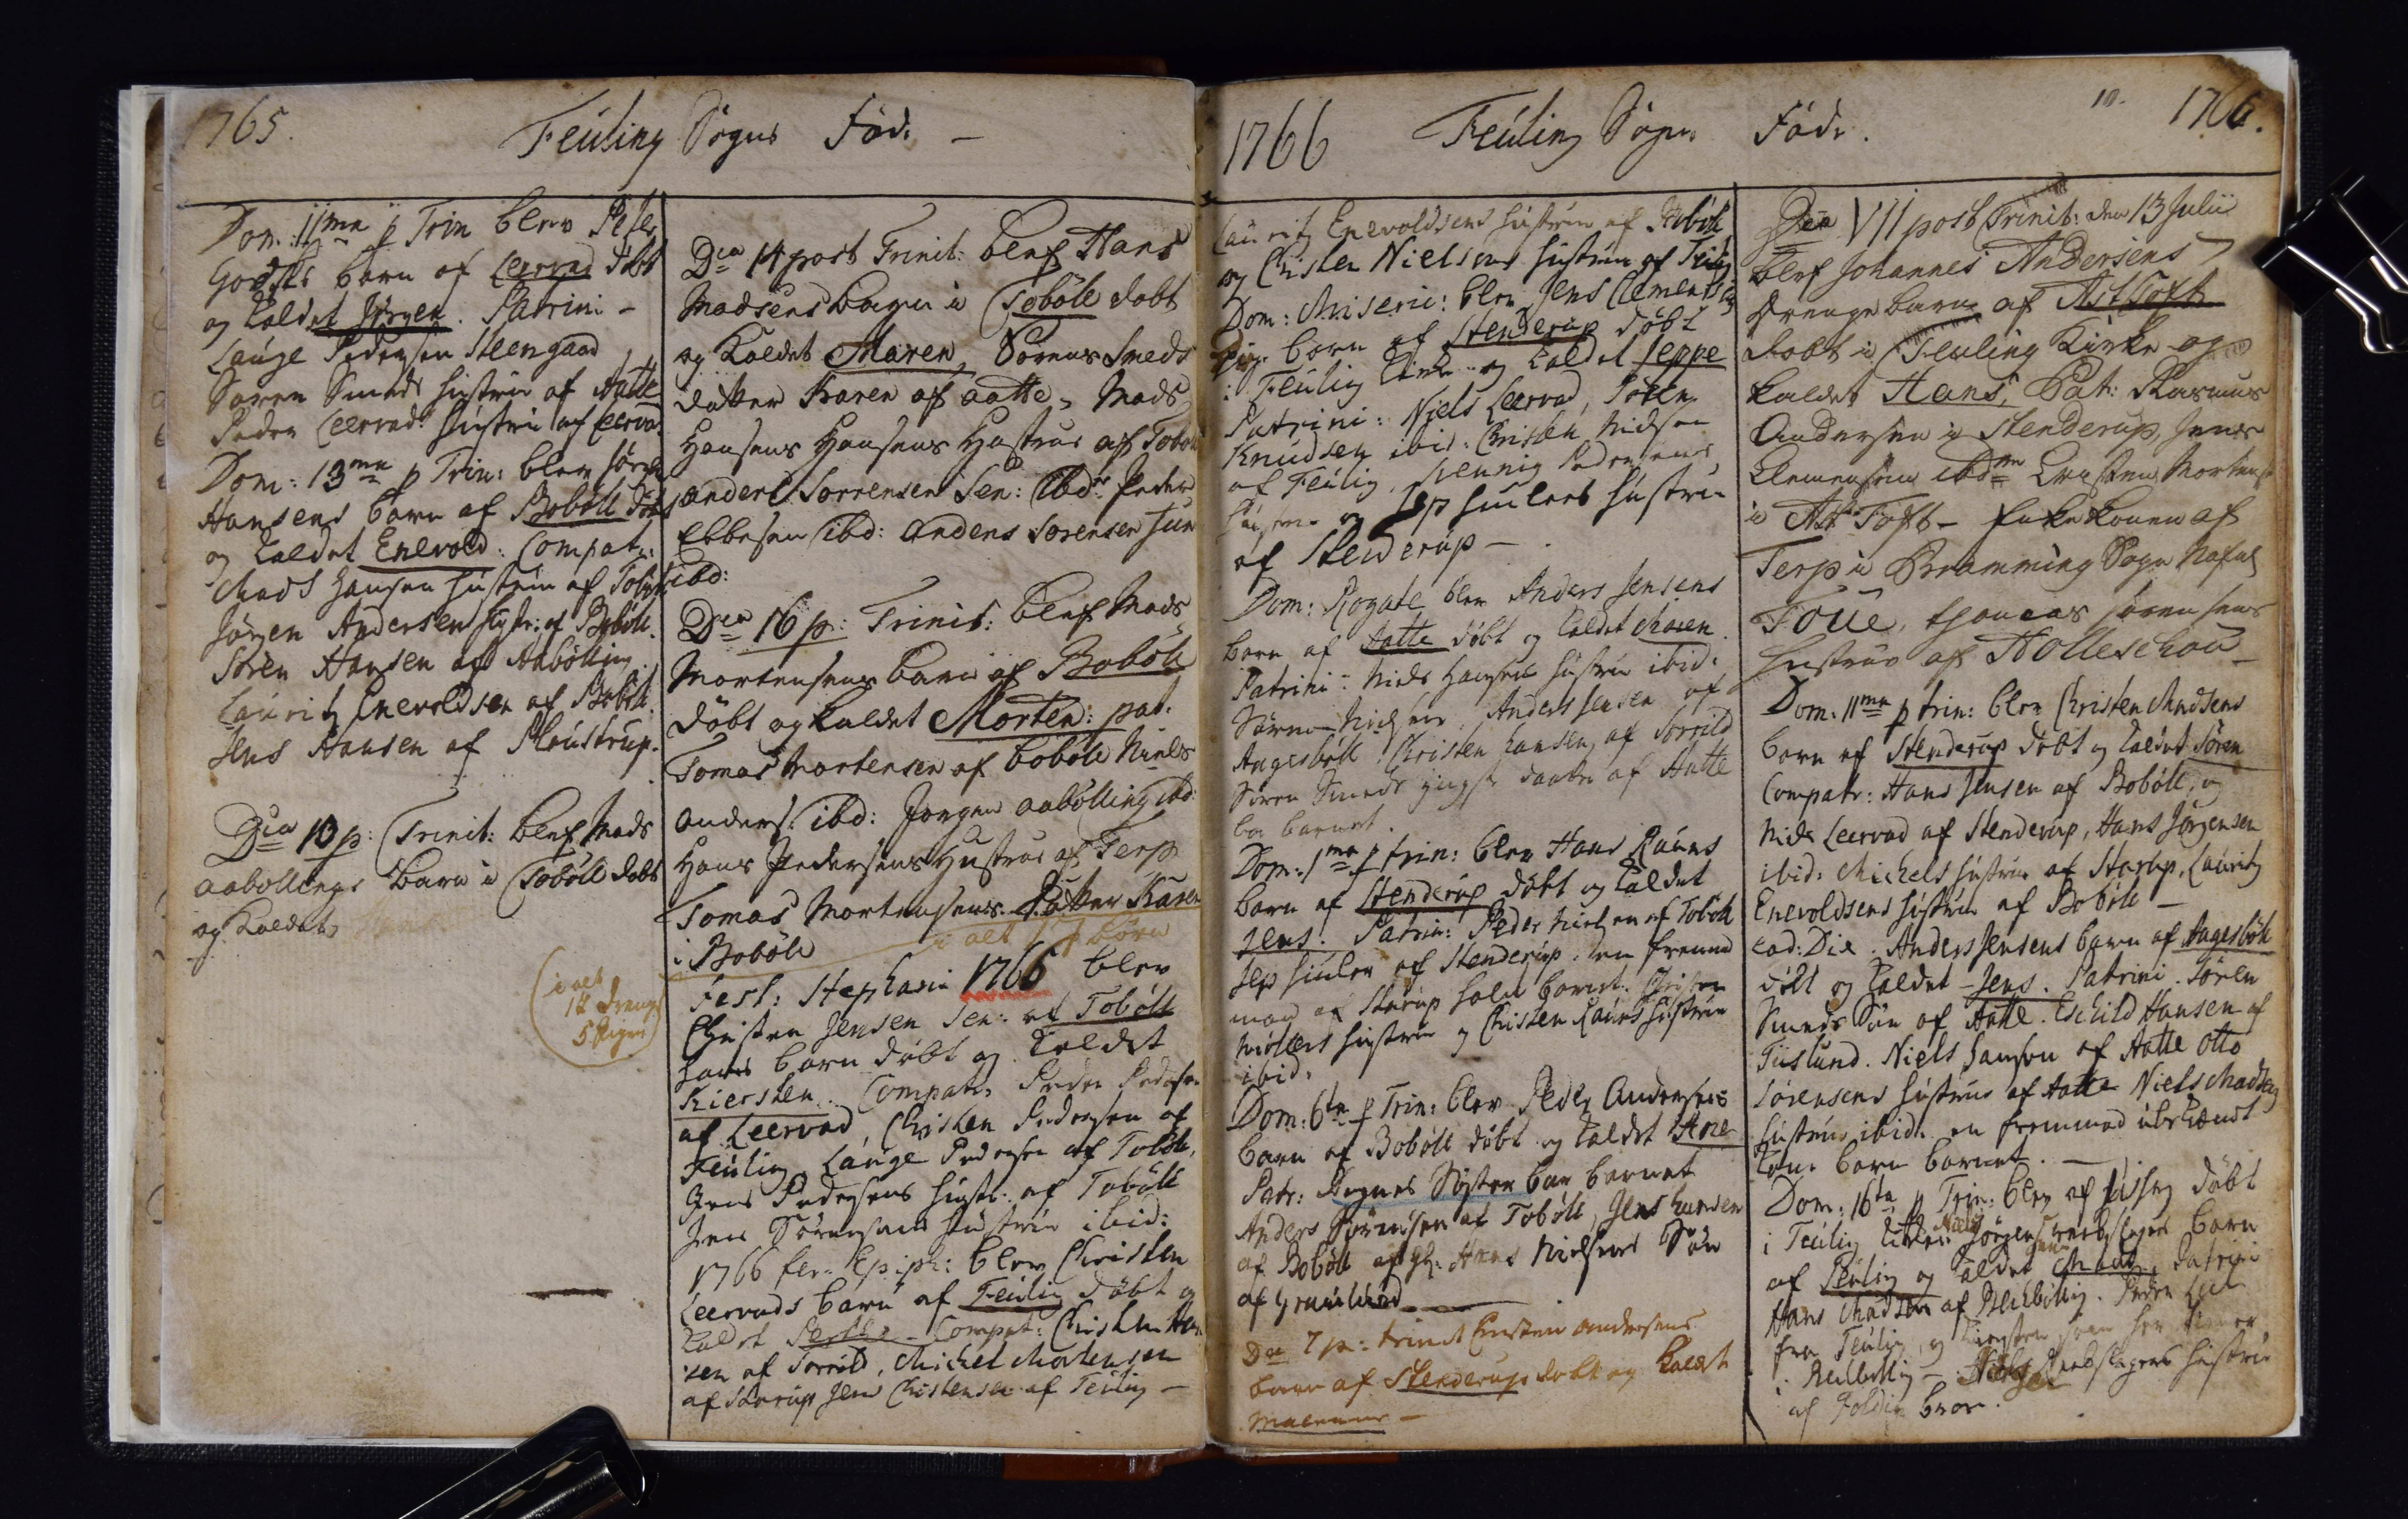1765.
Feuling Sogns Fød:
Dom: 11ma p Trin blev Peter
Godsks Barn af Leervad døbt
og kaldet Jørgen. Patrini -
Lauge Pedersen Steengaard
Søren Smeds hustru af Aatte
Peder Leervads hustru af Leervad
Dom: 13ma p Trin: blev Jørgen
Hansens barn af Bobøll døbt
og kaldet Enevold: Compat:
Mads hansen hustrue af Tobøl
Jørgen Andersens hustr: af Bobøll.
Søren Hansen af Aabølling
Lauritz Enevoldsen af Bobøl
Jens Hansen af Ploustrup.
Dca 10 p: Trinit: blef Mads
Aabøllings barn i Tobøll døbt
og kaldet
i alt
17 Drenge
5 Piger
Dca 14 post Trinit: blef Hans
Madsens barn i Tobøll dobt
og kaldet Maren, Sørens Smeds
datter Karen af Aatte, Mads
Hansens Hansens Hustrue af Toboll
Anders Sørrensen Sen: ibd## Peder
Ebbesøn ibd: Anders Sørensen Jun
ibd:
Dca 16 p: Trinit: blef Mads
Mortensens barn af Bobøll
døbt og kaldet Morten: pat.
Tomas Mortensen af bobøll Niels
Anders: ibd: Jørgen Aabølling ibid:
Hans Pedersens Hustrue af Terp
Tomas Mortensens Datter Karen
i Bobøl.
i alt 17 Børn
Fest: Stephani 1766 blev
Christen Jensen Sen: af Tobøll
hans barn døbt og kaldet
Kiersten. Compatr, Peder Pedersen
af Leervad, Christen Pedersen af
Feuling, Lauge Pedersen af Tobøll,
Jens Pedersens Hustr: af Tobøll
Jens Sørrensens Hustrue ibid:
1766 Fer: Epiph: blev Christen
Leervads barn af Feuling døbt og
kaldet ##rth## Compat: Christen Han¬
sen af Sorrild, Michel Mortensen
af Storup Jens Christensen af Feuling -
1766
Feuling Sogns Føde
Lauritz Enevoldsens hustrue af Bobøll
og Christen Nielsens Hustrue af Feuling
Dom: Miseric: blev Jens Clemensens
Pige Barn af Stenderup døbt
i Feuling Kirke og kaldet Jeppe
Patrini: Niels Leervad, Søren
Knudsen ibid. Christen Nielsen
af Feuling, Hennig Pedersens
hustru og Jep hiulers hustru
af Stenderup -
Dom: Rogate blev Anders Jensens
Barn af Aatte døbt og kaldet Maren
Patrini: Niels Hansens hustru ibid:
Søren Nielsen Anders Jensen af
Aagesbøll Christen Hansen af Sorrild
Søren Smeds Hustr daatter af Aatte
bar barnet.
Dom: 1ma p Trin: blev Hans Rauns
Barn af Stenderup døbt og kaldet
Jens. Patrin: Peder Nielsen af Tobøll
Jep hiulers af Stenderup. en fremmed
mand af Starup holdt barnet. Christen
Nielsens hustrue og Christen Rauns hustrue
ibid:
Dom. 6ta p Trin: blev Peder Andersens
Barn af Bobøll døbt og kaldet Ane
Patr: Degnes Søster bar barnet
Anders Sørensen af Tobøll, Jens Larsen
af Bobøll af gl: Hans Nielsøns Søn
af Graulund.
Dom: 7 p: Trinit Christen Andersens
barn af Stenderup døbt og kaldet
Maleene
10.
1766.
Dca VII post Trinit: d 13 Julii
blef Johannes Andersens
Drengebarn af Astoft
døbt i Feuling Kirke og
kaldet Hans Pat: Rasmus
Anderssen i Stenderup, Jens
Clemensen ibdm Kristen Mortensen
i Astoft. Enke Konen af
Terp i Bramming Sogn Nafnl
Toue, Thomas Sørensens
Hustrue af Holleschou
Dom: 11ma p Trin: blev Christen Madsens
Barn af Stenderup døbt og kaldet Søren
Compatr: Hans Jensen af Bobøll, og
Niels Leerved af Stenderup, Hans Jørgensen
ibid: Michels Hustrue af Starup, Lauritz
Enevoldsens Hustrue af Bobøll -
Eod: Die: Anders Jensens barn af Aagesbøll
døbt og kaldet - Jens. Patrini. Sørn¬
Smeds Søn af Aatte Eschild Hansen af
Tiislund. Niels Hansen af Aatte Otto
Sørensens hustrue af Aatte Niels Madsens
Hustrue ibid. en fremmed ubekiændt
kone barn barnet.
Dom: 16ta p Trin: blev af ## døbt
Niels
i Feuling Kirke Jørgen reebslagers barn
Jens
af Feuling og kaldet Mads Patrini
Hans Madsen af Bechbølling. Peder L##
fra Feuling, og Kiersten ## her ##
i Bechbølling - Jørgen Reebslagers hutrue
af Folding broe
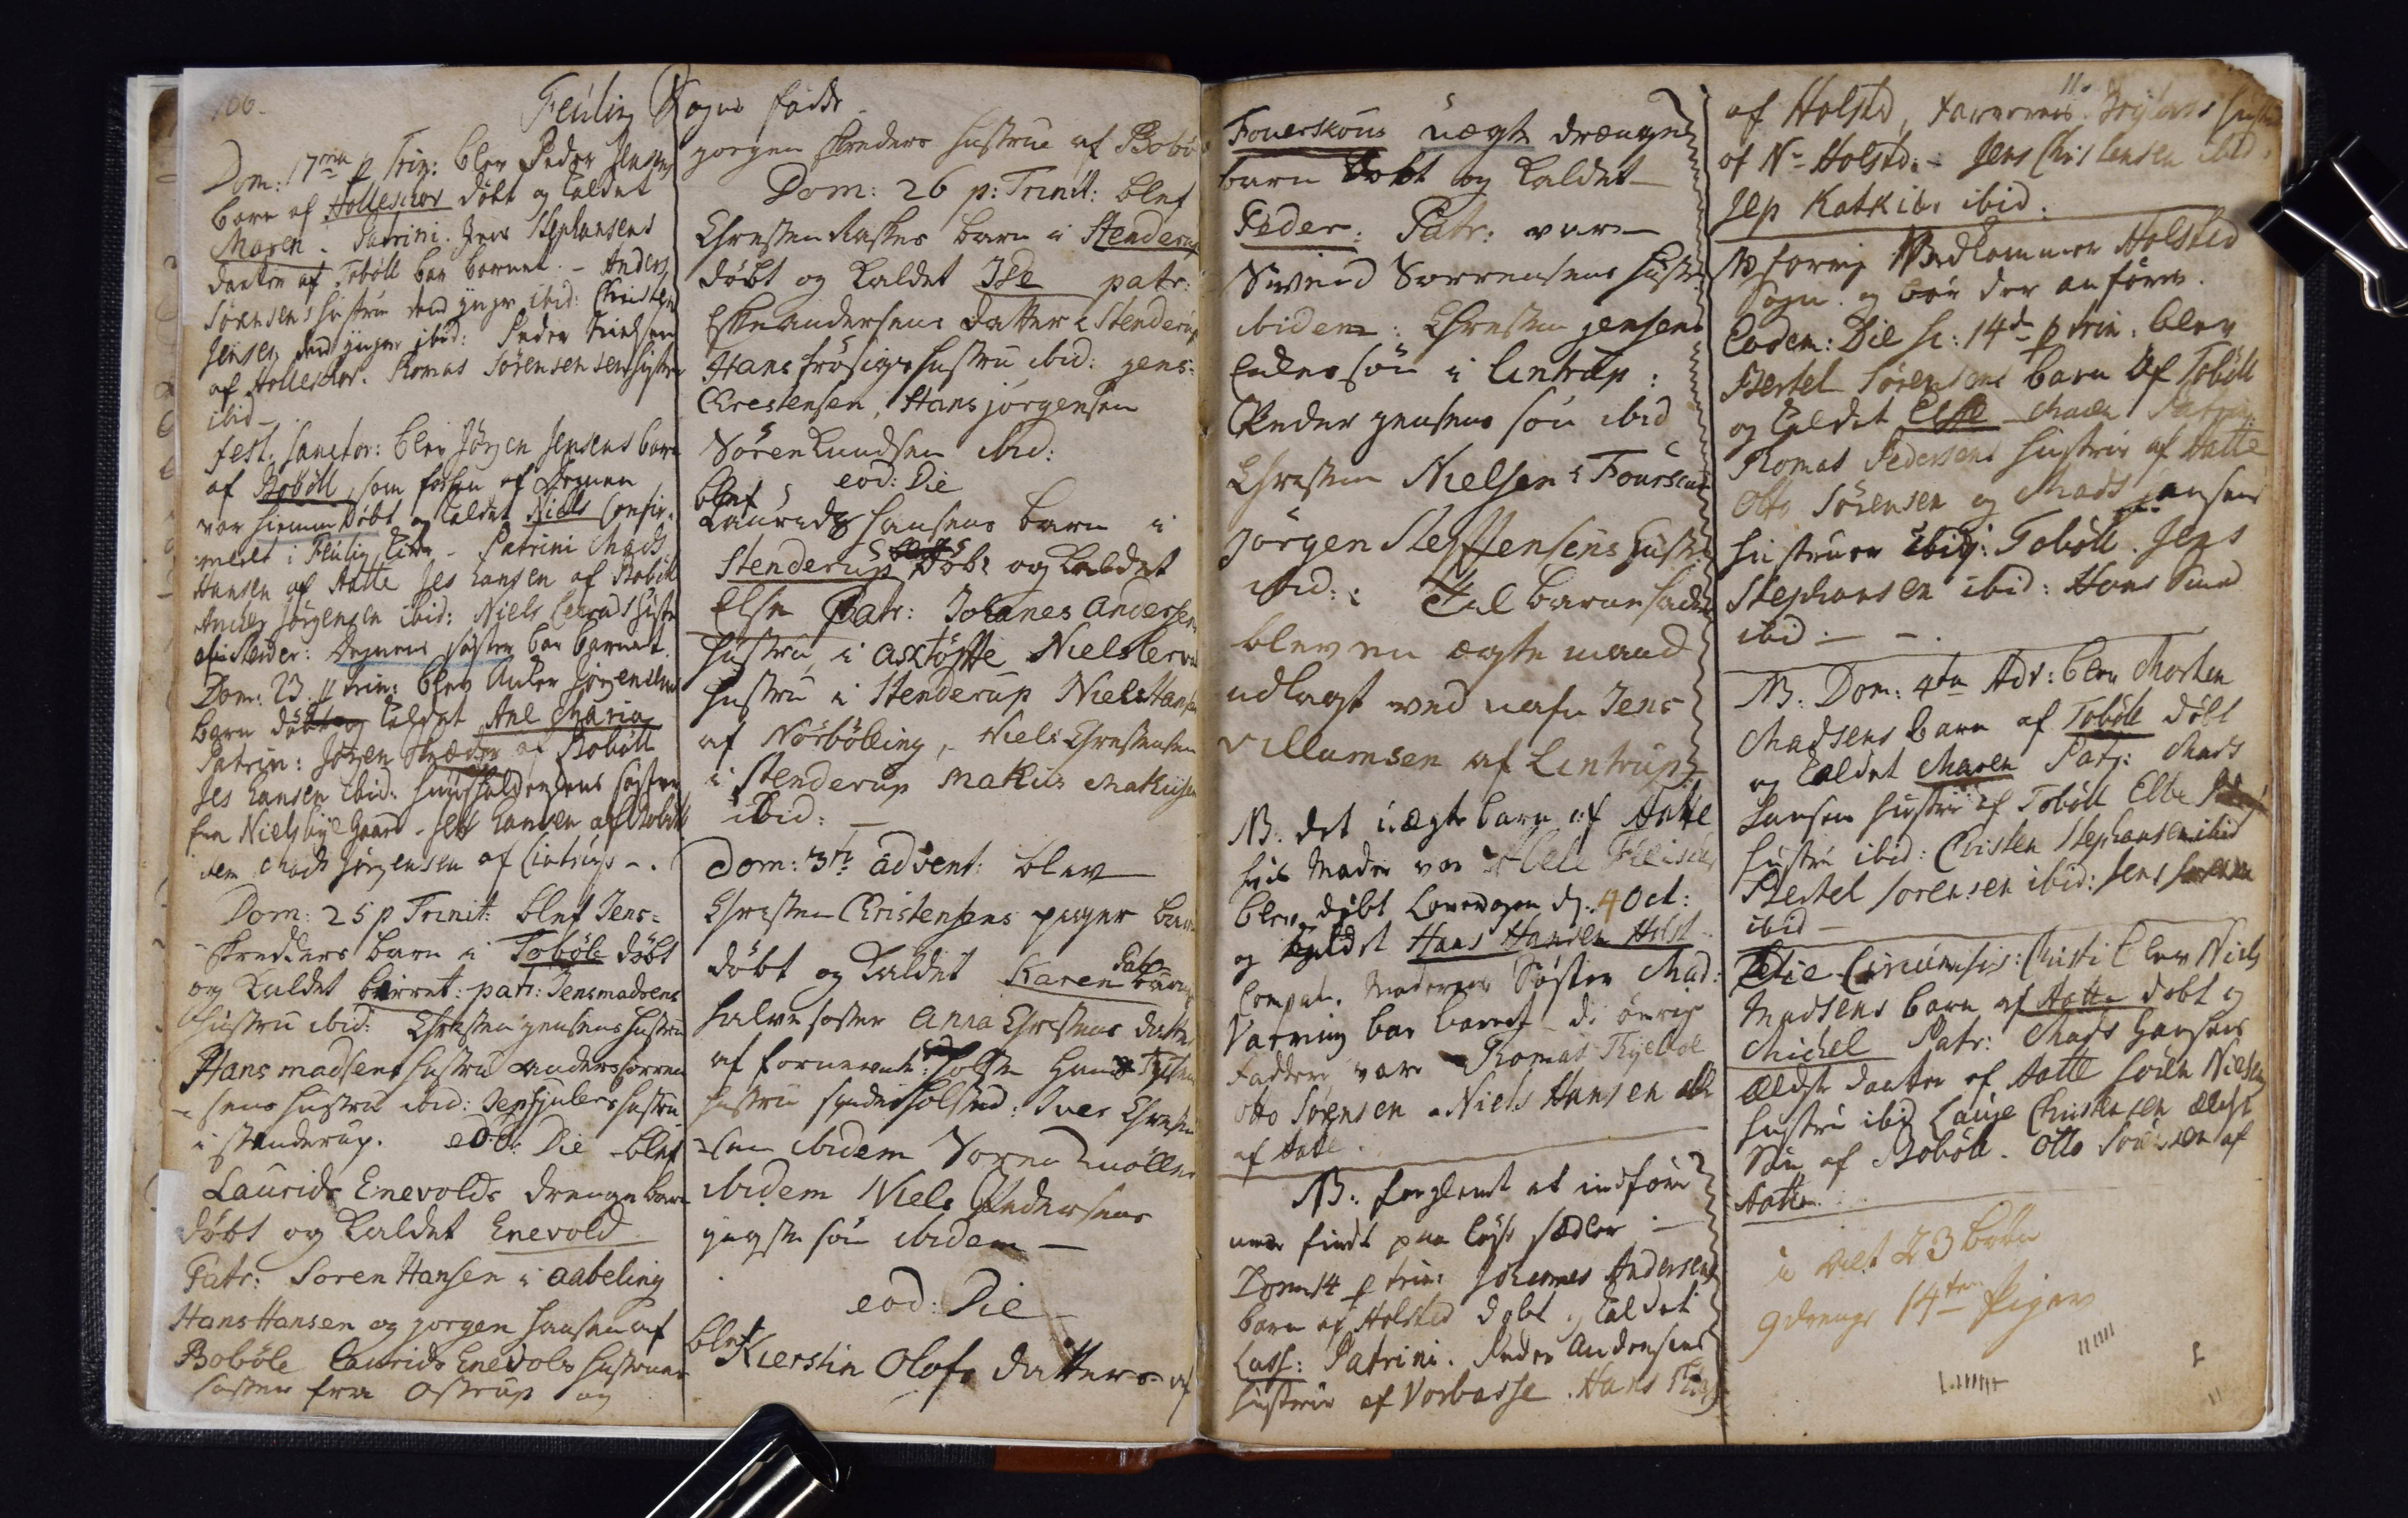106
Feuling Sogns fødde
Dom: 17ma p Trin: blev Peder Hansen
barn af Holleschov døbt og kaldet
Maren. Patrini. Jens Stephansens
daatter af Tobøll bar barnet. - Anders
Sørensens hustrue dend yngre ibid: Christen
Jensen den yngre ibid: Peder Nielsens
af Holleskov. Tomas Sørensen Sen Hustru
ibid -
Fest. Sanctor: blev Jørgen Jensens barn
af Bobøll, som forhen af Degen
var hiemmedøbt og kaldet Niels Confir¬
meret i Feuling Kirke - Patrini Mads
Hansen af Aatte Jes Hansen af Bobøll
Ancher Jørgensen ibid: Niels Leervads hustr
af Stender: Degnens Søster bar Barnet.
Dom: 23. p Trin: blev Anker Jørgensens
barn døbt og kaldet Ane Maria
Patrin: Jørgen Skræder af Bobøll
Jes Hansen ibid: huusholderens søster
fra Nielsbye Gaard Jes Hansen af Bobøll
item Mads Jørgensen af Lintrup - .
Dom: 25 p Trinit: blef Jens
Skredders barn i Tobøl døbt
og kaldet birret: patr: Jens madsens
Hustru ibid: Christen Jensens Hustru
Hans Madsens Hustru Anders Sørren
sens Hustru ibid: Jep hjulers hustru
i Stenderup. Eod Die blef
Laurids Enevolds Drenge barn
døbt og kaldet Enevold
Patr: Søren Hansen i Aabeling
Hans Hansen og Jørgen Hansen af
Bobøle, Laurids Enevols Hustrue
Soster fra Ostrup og
Jørgen Skreders Hustru af Bobø 
Dom: 26 p: Trinit: blef
Christen Raskes Barn i Stenderup
døbt og kaldet Ide patr:
Eske Andersens Datter i Stenderup
Hans Frøsigs Hustru ibid: jens
Chrestensen, Hans jørgensen
Søren Knudsen ibid:
blef eod: Die
Laurids Hansens barn i
Stenderup døbt og kaldet
Else Patr: Johannes Andersens
Hustru i Asstøftte Niels Lervad 
Hustru i Stenderup Niels Hansen
af Nørbølling, Niels Christensen
i Stenderup Mathiis Mathiisen
ibid:
Dom: 3## Advent: blev
Christen Christensens piger barn
Patr:
døbt og kaldet Karen Barn##
halve søster Anna Christens datter
af fornævnte ##olste Hans T##
Hustru synderholsted: Iver Christen
sen ibidem Søren Møller
ibidem Niels Pedersens
yngste søn ibidem
eod: Die
blef: Kierstin Olofs datters af
Fouerskous  uægte drænge
barn døbt og kaldet
Peder: Patr: var
Smed Sørrensens Hustr
ibidem:  Christen jensens
Enkes søn i lintrup
Peder Jensens søn ibid.
Christen Nielsen i Foursk##
Jørgen Steffensens Hustr:
ibid: Til Barnefader
blev en ægtemand
udlagt ved nafn Jens.
Villumsen af Lintrup.
NB. det uægte barn af Aatte
hvis Moder var Abele Fl##
blev døbt Lovedagen d: 4 Oct:
og kaldet Hans Hansen Holst
Compat: Moderens Søster Mad
Varming bar barnet d: øvrige
Faddere vare Thomas Thyebol
Otto Sørensen Niels Hansen alle
af Aatte
NB: forglemt at indfor
mere findt paa løse sædler
Dom 14 p Trin: Johannes Andersen
barn af Holsted døbt kaldet
Lass: Patrini. Peder Andersens
Hustru af Vorbvasse, Hans Chr  
11
af Holsted. Fa## ## Hus   
af N= Holsted. Jens Christensen ibid.
Jep Katkibs ibid
toforige Vedkommer Holsted
Sogn og bør der anføres.
Eodem Die d: 14de p Trin. blev
Bertel Sørensens barn af Tobøll
og kaldet Else Madz ##:
Thomas Pedersens Hustrue af Aatte
Otte Sørensen og Mads Hansens
Hustruer ibid: Tobøll. Jens
Stephansen ibid: Hans Smed
ibid:
NB. Dom: 4ta Adv: blev Morten
Madsens Barn af Tobøll døbt
og kaldet Maren Patr: Mads
Larsens hustrue af Tobøll Elbe Pedersens
hustru ibid: Christen Stephansen ibid.
Bertel Sørensen ibid: Jens Hansen
ibid:
##tie Circumsis: Christi blev Niels
Madsens barn af Aatte døbt og
Michel Patr: Mads Hansens
ældste daatter af Aatte søren Nielsens
Hustru ibd. Lauge Christensen ælste
Søn af Bobøll. Otto Søn en aff
Aatte.
i alt 23 Børn
9 drenge 14ten Piger.